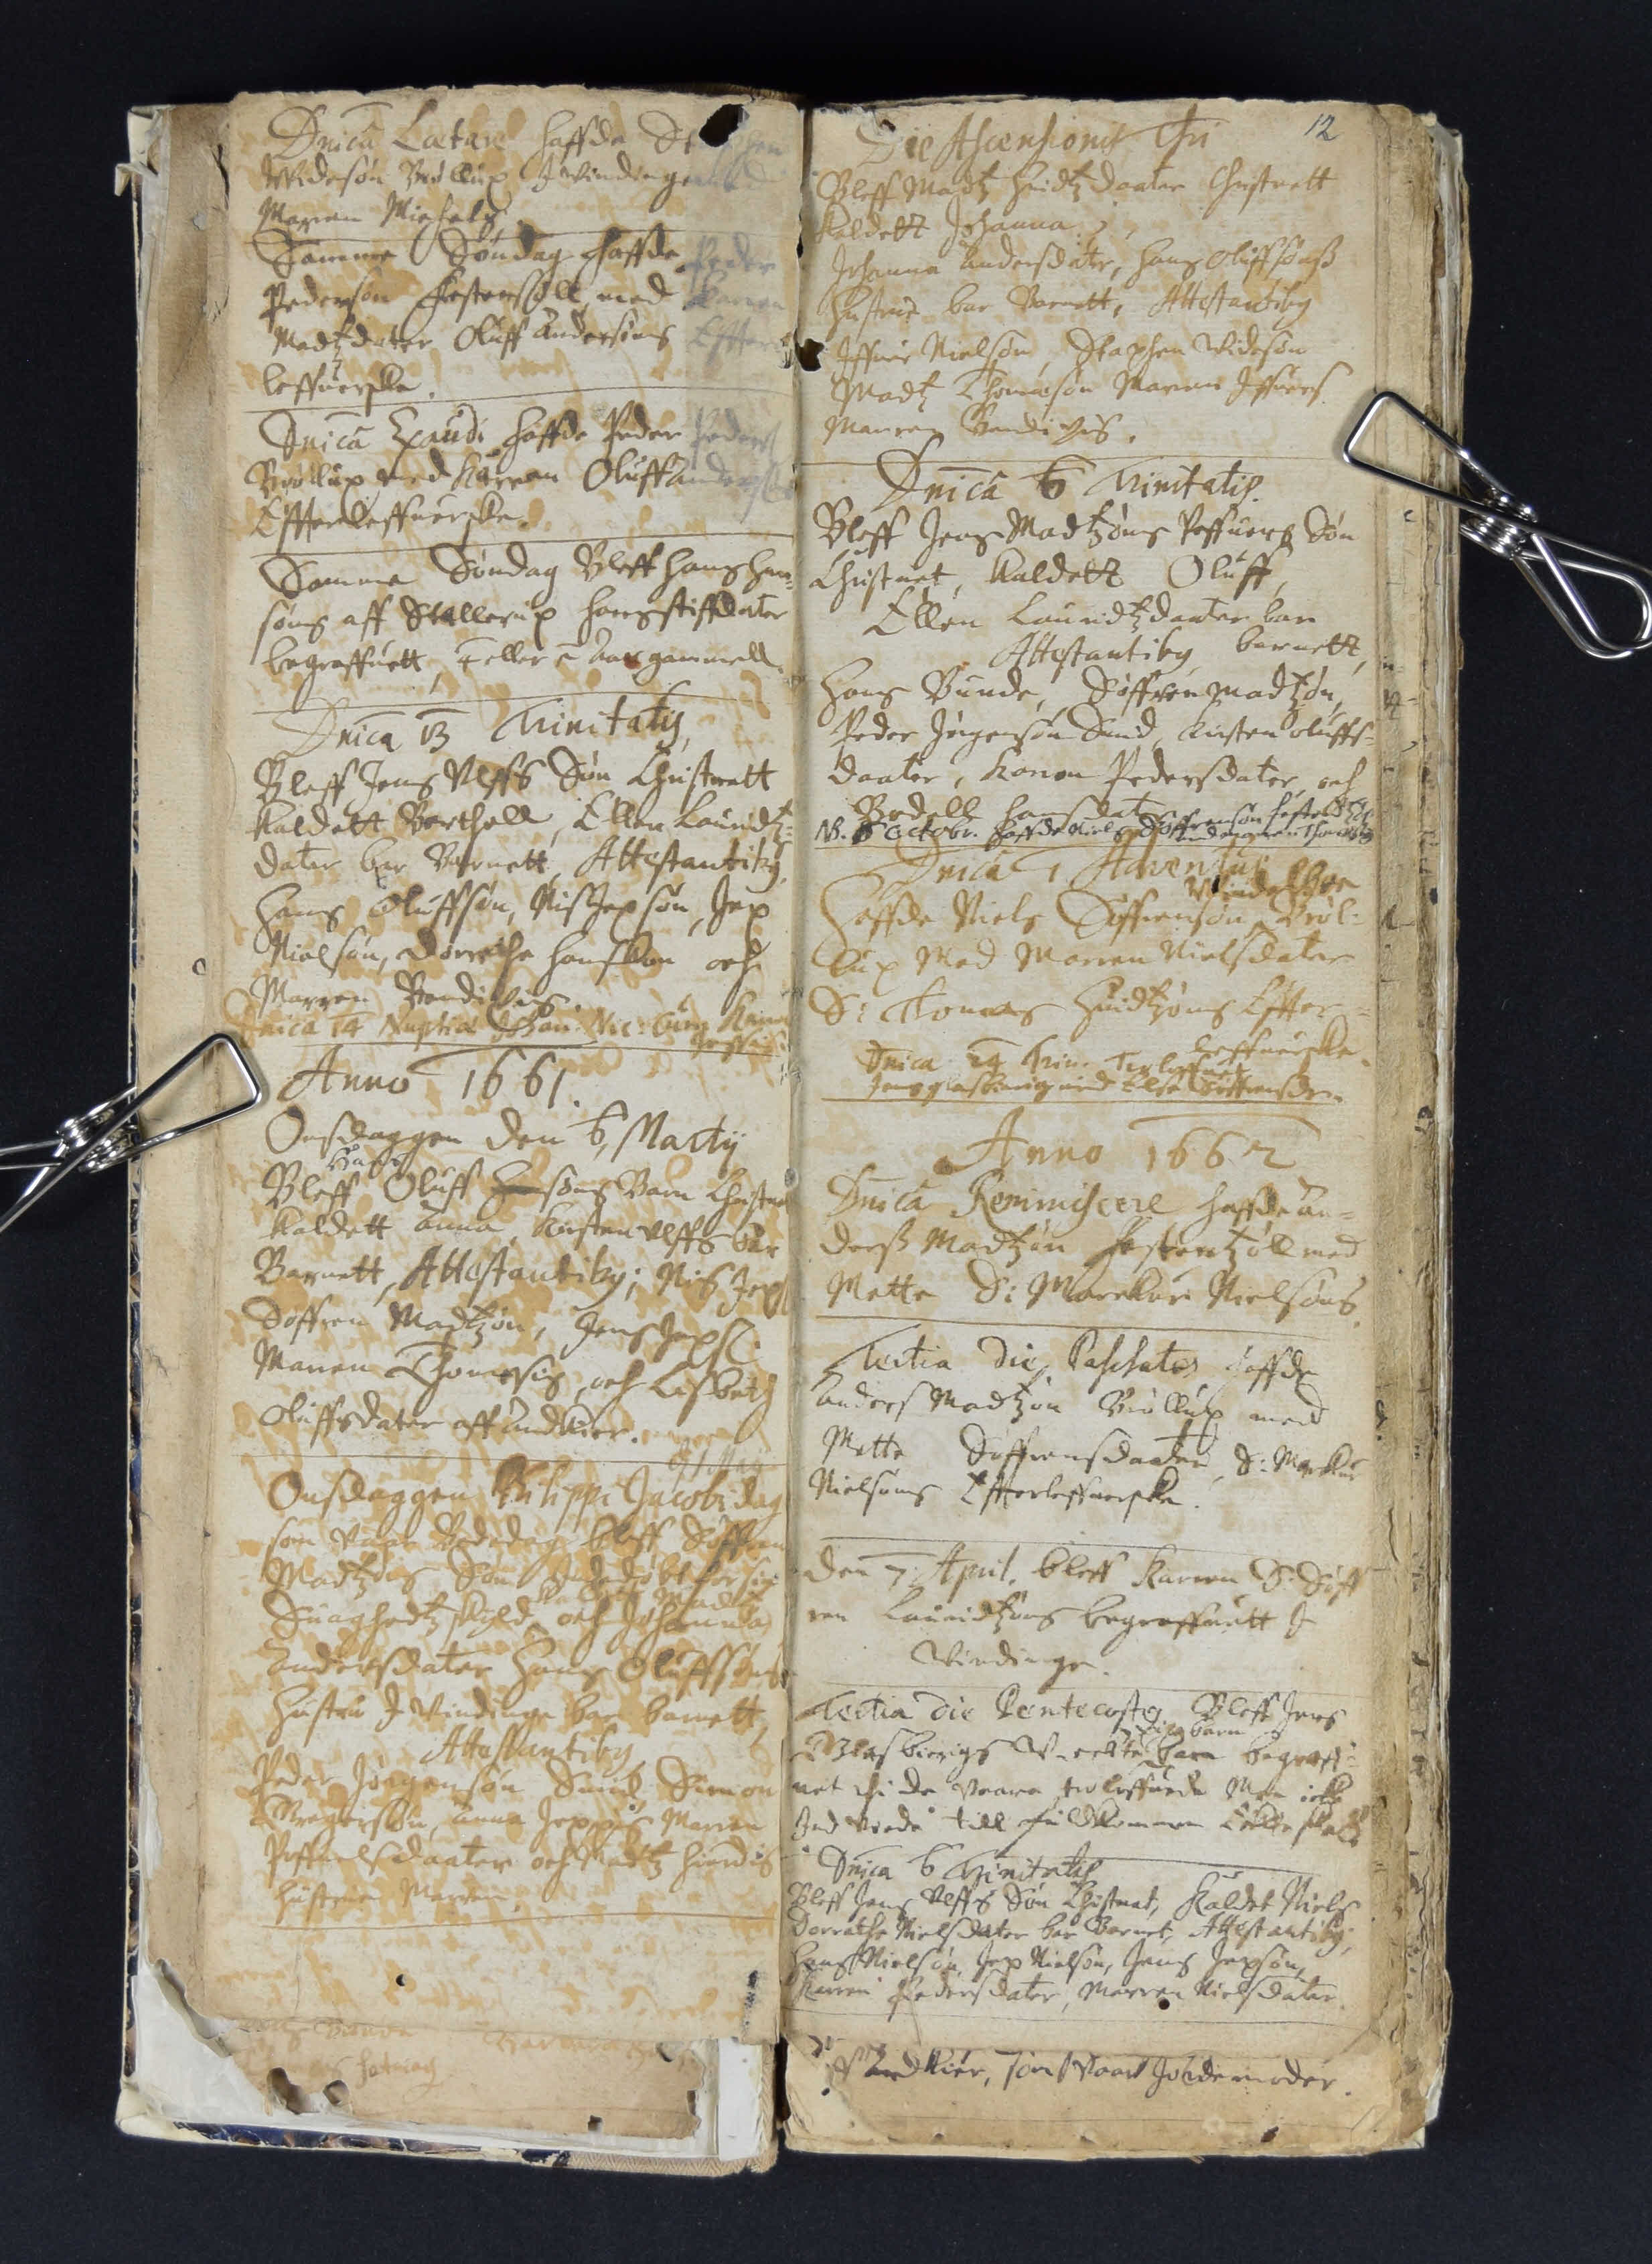Dnica Latare haffde
Widesøn Brøllup I Windinge
Maren Michels
Samme Søndag Haffde Peder
Pedersøn Festens øll med Karen 
Madtzdater Oluff Andersøns Effter¬
leffuerske
Dnica Exaudi. Haffde Peder Peders
Brøllup med Karren Oluff Anders
Efeterleffuerske.
Samme Søndag Bleff Hans Han¬
søns aff Stallerup hans stiffdater
begraffuett 4 eller 5 Aar gammell.
Dnica 13 Trinitatis
Bleff Jens Vlffs Søn Christnett
kaldett Berthell, Ellen Lauridtz¬
dater bar Barnet, Attestantiby.
Hans Oluffsøn. Nis Jepsøn. Jep
Nielsøn. Dorethe Hans Kon och
Marren Bendixsis
Dnica 14. Nuptia Johan Nic:
Jessis.
Anno 1661.
Onsdaggen den 6 Martij
Hans
Bleff Oluff H##søns Barn Christnet
kaldett Anna, Kirsten Vlffs bar
Barnet. Attestantiby; Nis Jeps
Søffren Madtzøn, Jens Jeps.
Maren Thomasis, och Lisbeth
Oluffsdater aff Andkier.
Onsdaggen Philippe Jacobs dag
som vaare Bededag bleff Søffren
Madtzøns Søn Indedøbt for sin
kaldett Madtz
suaghedtz skyld och Johanna
Andersdater Hans Oluffsøns
Hustrue I Vindinge bar barnet,
Attestantiby
Peder Jørgensøn Smid, Simon
Gregersøn, Anna Jeppis Maren
Poffuelsdaater och Madtz Hiurdis
Hustrue Marren.
12
Die Asansionis Chri
Bleff Madtz Hvidtz daater christnett
kaldett Johanna
Johanna Andersdater, Hans Oluffsønss
Hustrue bar Barnet. Attestantiby
Iffuer Nielsøn, Staphen Widesøn
Madtz Thomasøn Maren Iffuers.
Maren Bendixses.
Dnica 6 Trinitatis.
Bleff Jens Madtzøns Veffuers Søn
Christnet, kaldett Oluff
Ellen Lauridtzdaater bar
barnet,
Attestantiby
Hans Bunde, Søffren Madtzøn,
Peder Jørgensøn Smid, Kirsten Oluff¬
daater, Karen Pedersdater, och
Bodell Hansdater.
NB. 6 Octobr. Haffde Niels Søffrensøn festens
med Maren Thomasis
Dnica 1 Adventus.
Windelboe
Haffde Niels Søffrensøn Brøl¬
lup med Marren Nielsdater
S: Thomas Hvidtzøns Efter¬
leffuerske
Dnica 24 Trin. Troloffuet
Jens Glasbierg med Else Søffrensdr.
Anno 1662
Dnica Reminiscere. Haffde An¬
ders Madtzøn festentz øll med
Mette S: Marckus Nielsøns
Tertia die Paschates. Haffde
Anders Madtzøn Brøllup med
Mette Søffrensdaater S: Markus
Nielsøns Efterleffuerske
den 7 April. bleff Karren S: Søff
ren Lauridtzøns begraffuett I
Vindinge.
Tertia die Pentecost. Bleff Jens
barn
Blasbiergs Ueekte barn begraff¬
uet thi de vaare troluffuede men icke 
Ind viede till fullkommen Eckeskab
Dnica 6 Trinitatis
Bleff Jens Vlffs Søn Christnet, kaldet Niels
Dorrethe Nielsdater bar Barnet. Attestantiby;
Hans Nielsøn, Jep Nielsøn, Jens Jensøn,
Karren Pedersdater, Marren Nielsdater